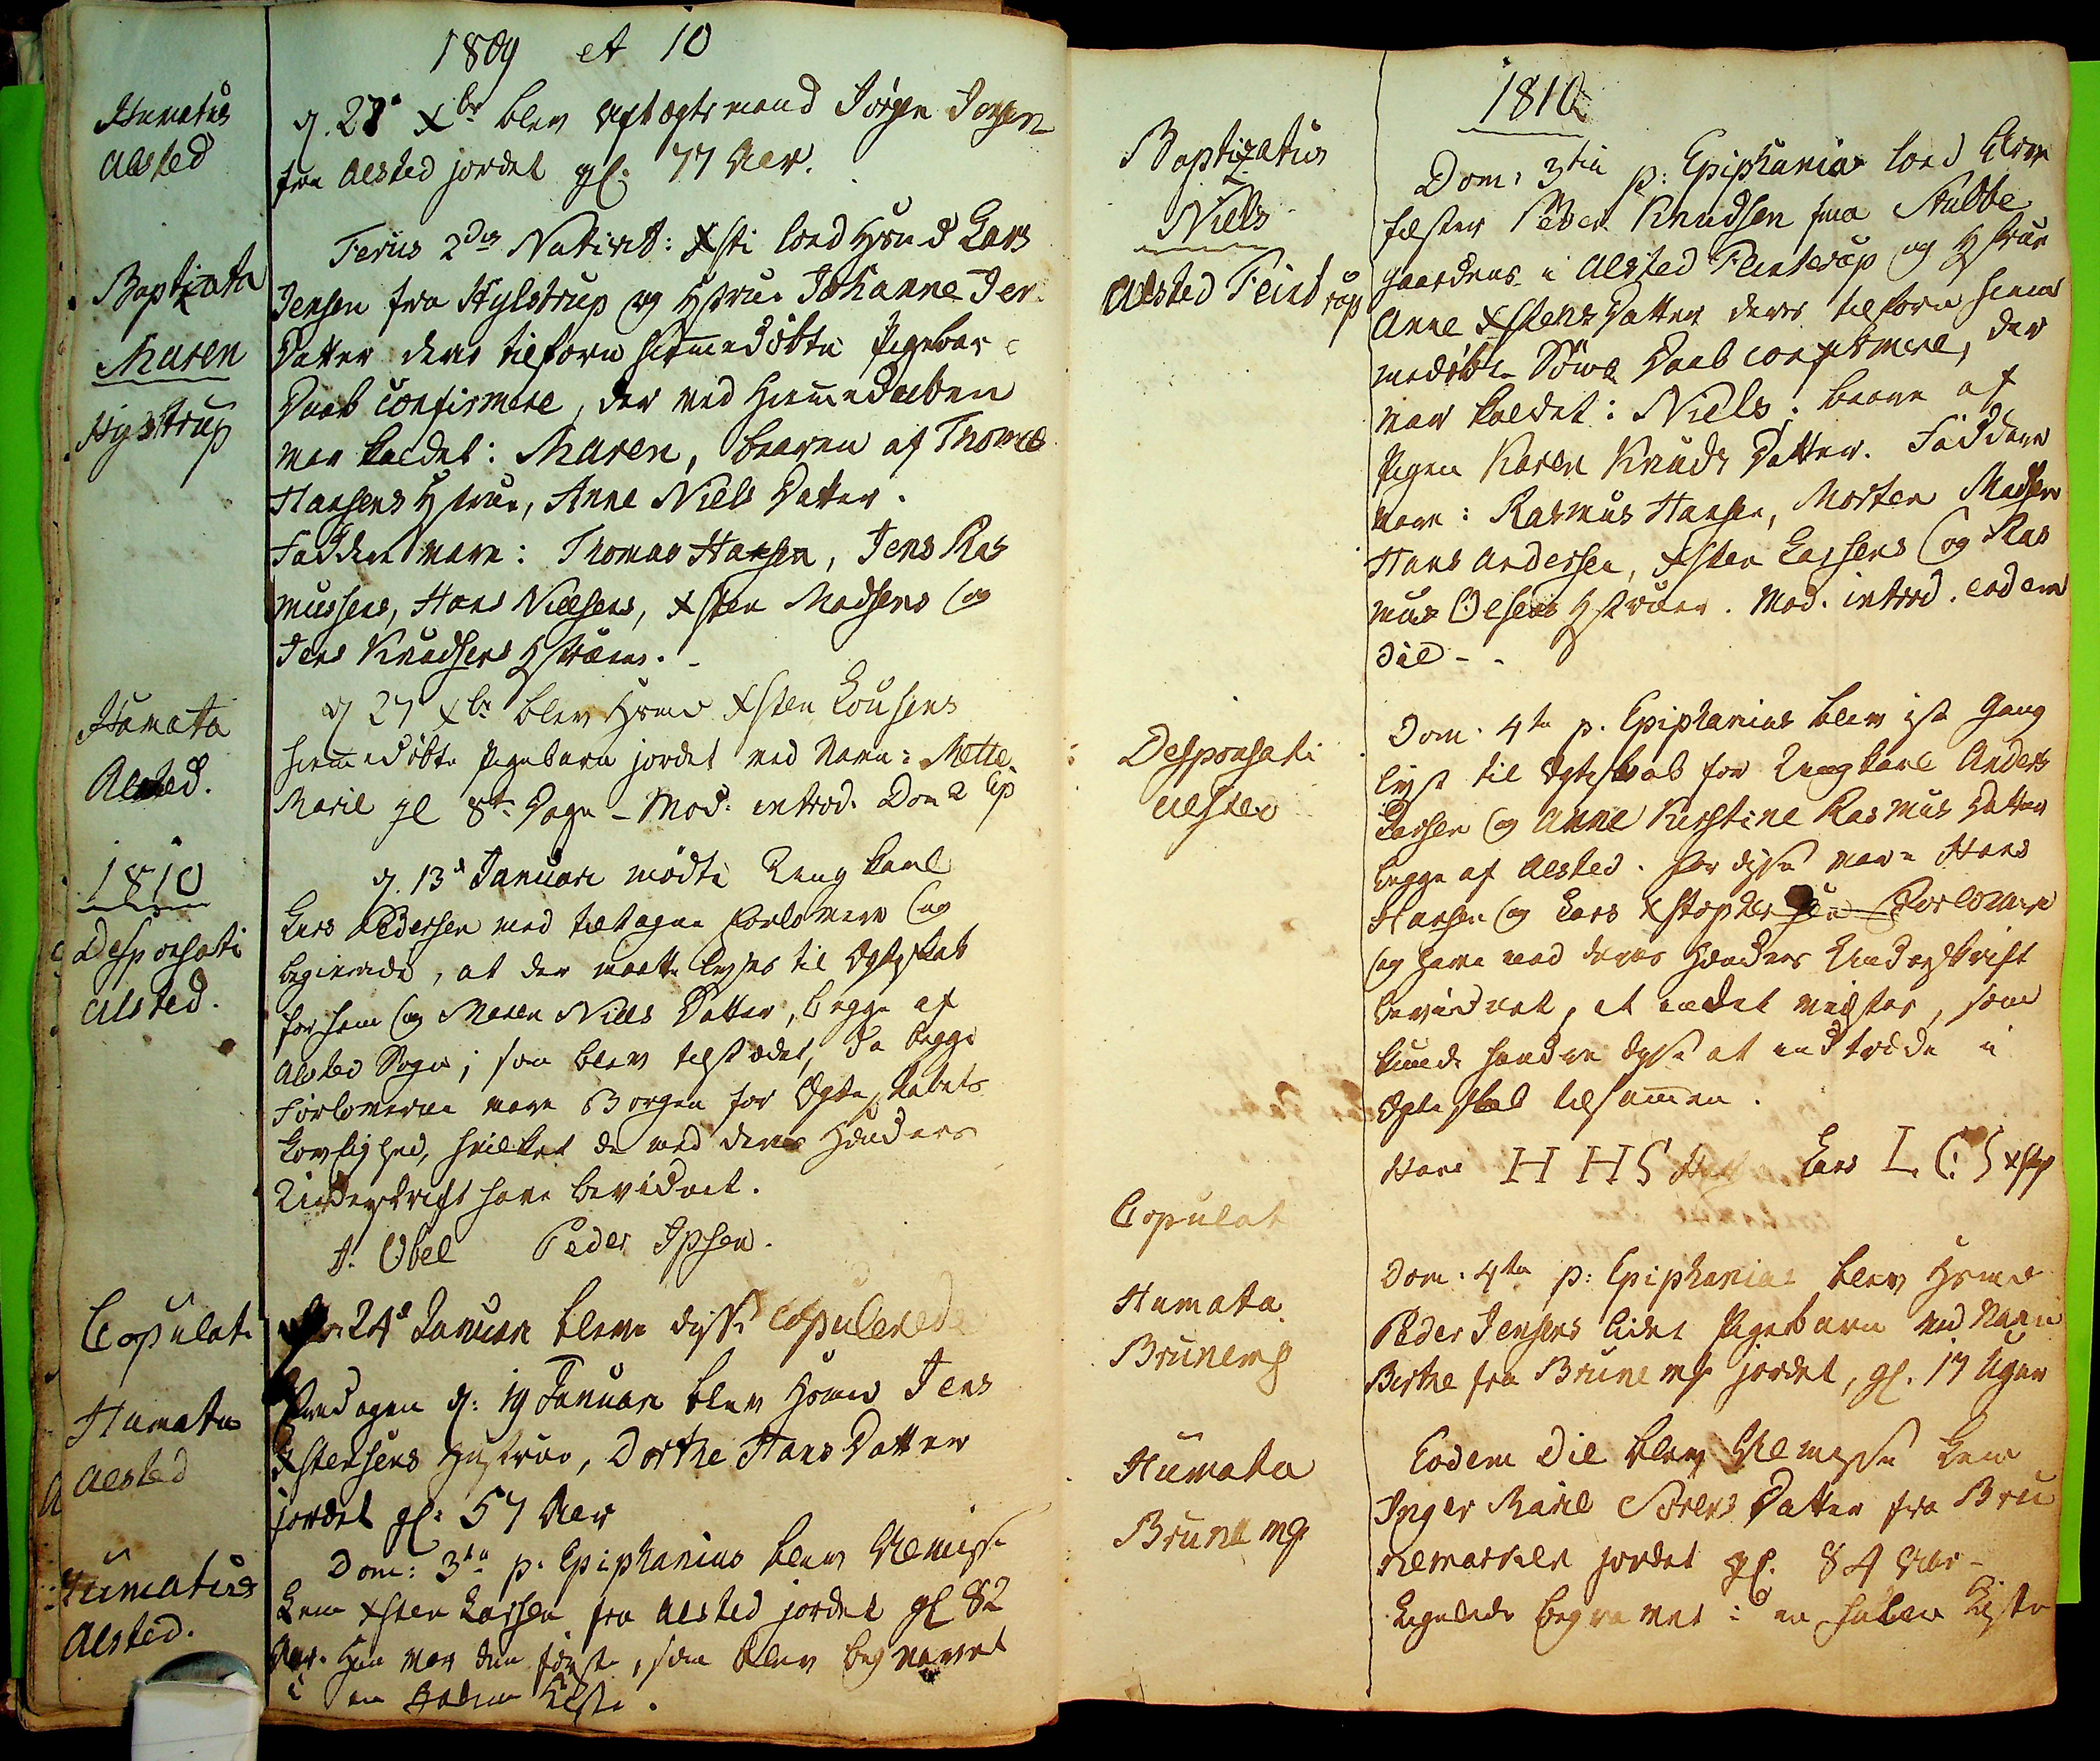Humatus
Alsted
Baptizata
Maren
Hylstrup
Humata
Alsted.
1810
Desponsati
Alsted
Copulati
Humata
Alsted
Humatus
Alsted
1809 et 10
d 27 Xbr blev Aftægtsmand Jørgen Jensen
fra Alsted jordet gl: 77 Aar.
Feriis 2den Nativt: Xsti leod Hsmd Lars
Jensen fra Hylstrup og Hstru Johanne Je##
Datter dere tillforn hiemmedøbte Pigebar##
Daab Confirmere, der ved Hiemmedaaben
var kaldet: Maren, baaren af Thomas
Hansens Hstruye, Anne Niels Datter.
Faddere vare: Thomas Hansen, Jens Ras¬
mussens, Hans Nielsens, Xsten Madsens og
Jens Knudsens Hstruer.
d 27 Xbr blev Hsmd Xsten Lousens
hiemedøbte Pigebarn jordet ved Navn: Mette
Marie gl 8te Dage - Mod: introd: Dom 2 Ep
d. 13de Januari mødte Ung m Karl
Lars Pedersen ved tiltagne Forlovere og
begierde, at der uægte lyses til Ægteskab
for ham og Maren Niels Datter, begge af
Alsted Sogn; som blev tilstædet da begge
Forlovere vare Borgen for Ægteskabets
Lovlighed, hvilket de ved dene Hænders
Underskrift have bevidnet.
J. Obel Peder Ipsen
## 24## Januar bleve disse copulerede
Fredagen d: 19 Januar blev Hsmd Jens¬
Xstensens Hustrue, Dorthe Hans Datter
Jordet gl: 57 Aar.
Dom: 3ta p. Epiphanias blev Almisse
Lem Xsten Larsen fra Alsted jordet gl 82
Aar Han den den første, som blev begravet
i en Ha## Kiste.
Baptizatus
Niels
Alsted Flinterup
Desponsati
Alsted
Copulat
Humata
Brunem##
Humata
Brunem##
1810
Dom: 3tia p: Epiphanias loed Arve
fæster Peder Knudsen paa Stubbe
Gaardens i Alsted Flinterup og Hstrue
Anne Xstens Datter deres tilforn hiem
medøbte Søns Daab confirmere der
var kaldet: Niels. baare af
Pigen Karen Knuds Datter. Faddere
vare: Rasmus Hansen, Morten Madsen
Hans Anderssen, Xten Larsens og Ras
mus Olsens Hstruer. Mod. introd: eodem
die -
Dom: 4ta p. Epiphanias blev 1st Gang
lyst til Ægteskab for Ungkarl Anders
Larsen og Anne Kirstine Rasmus Datter
begge af Alsted for disse vare Hans
Hansen og Lars Xstophersen Forlovere
og have ved deres Hænders Underskrift
bevidnet, at ##det vidstes, som
kunde hændre disse at indsræde i
Ægteskab tilsammen
Hans H H S Hansen Lars L C S Xstens
Dom: 4ta p: Epiphanias blev Hsmd
Peder Jensens lidet Pigebarn ved Navn
Birthe fra Brunem## jordet gl. 17 Uger
Eodem Die blev Almisse Lem
Inger Marie Sørens Datter fra Bru
nemarken jordet gl: 84 Aar.
Ligeledes begravet i en hal## Kiste
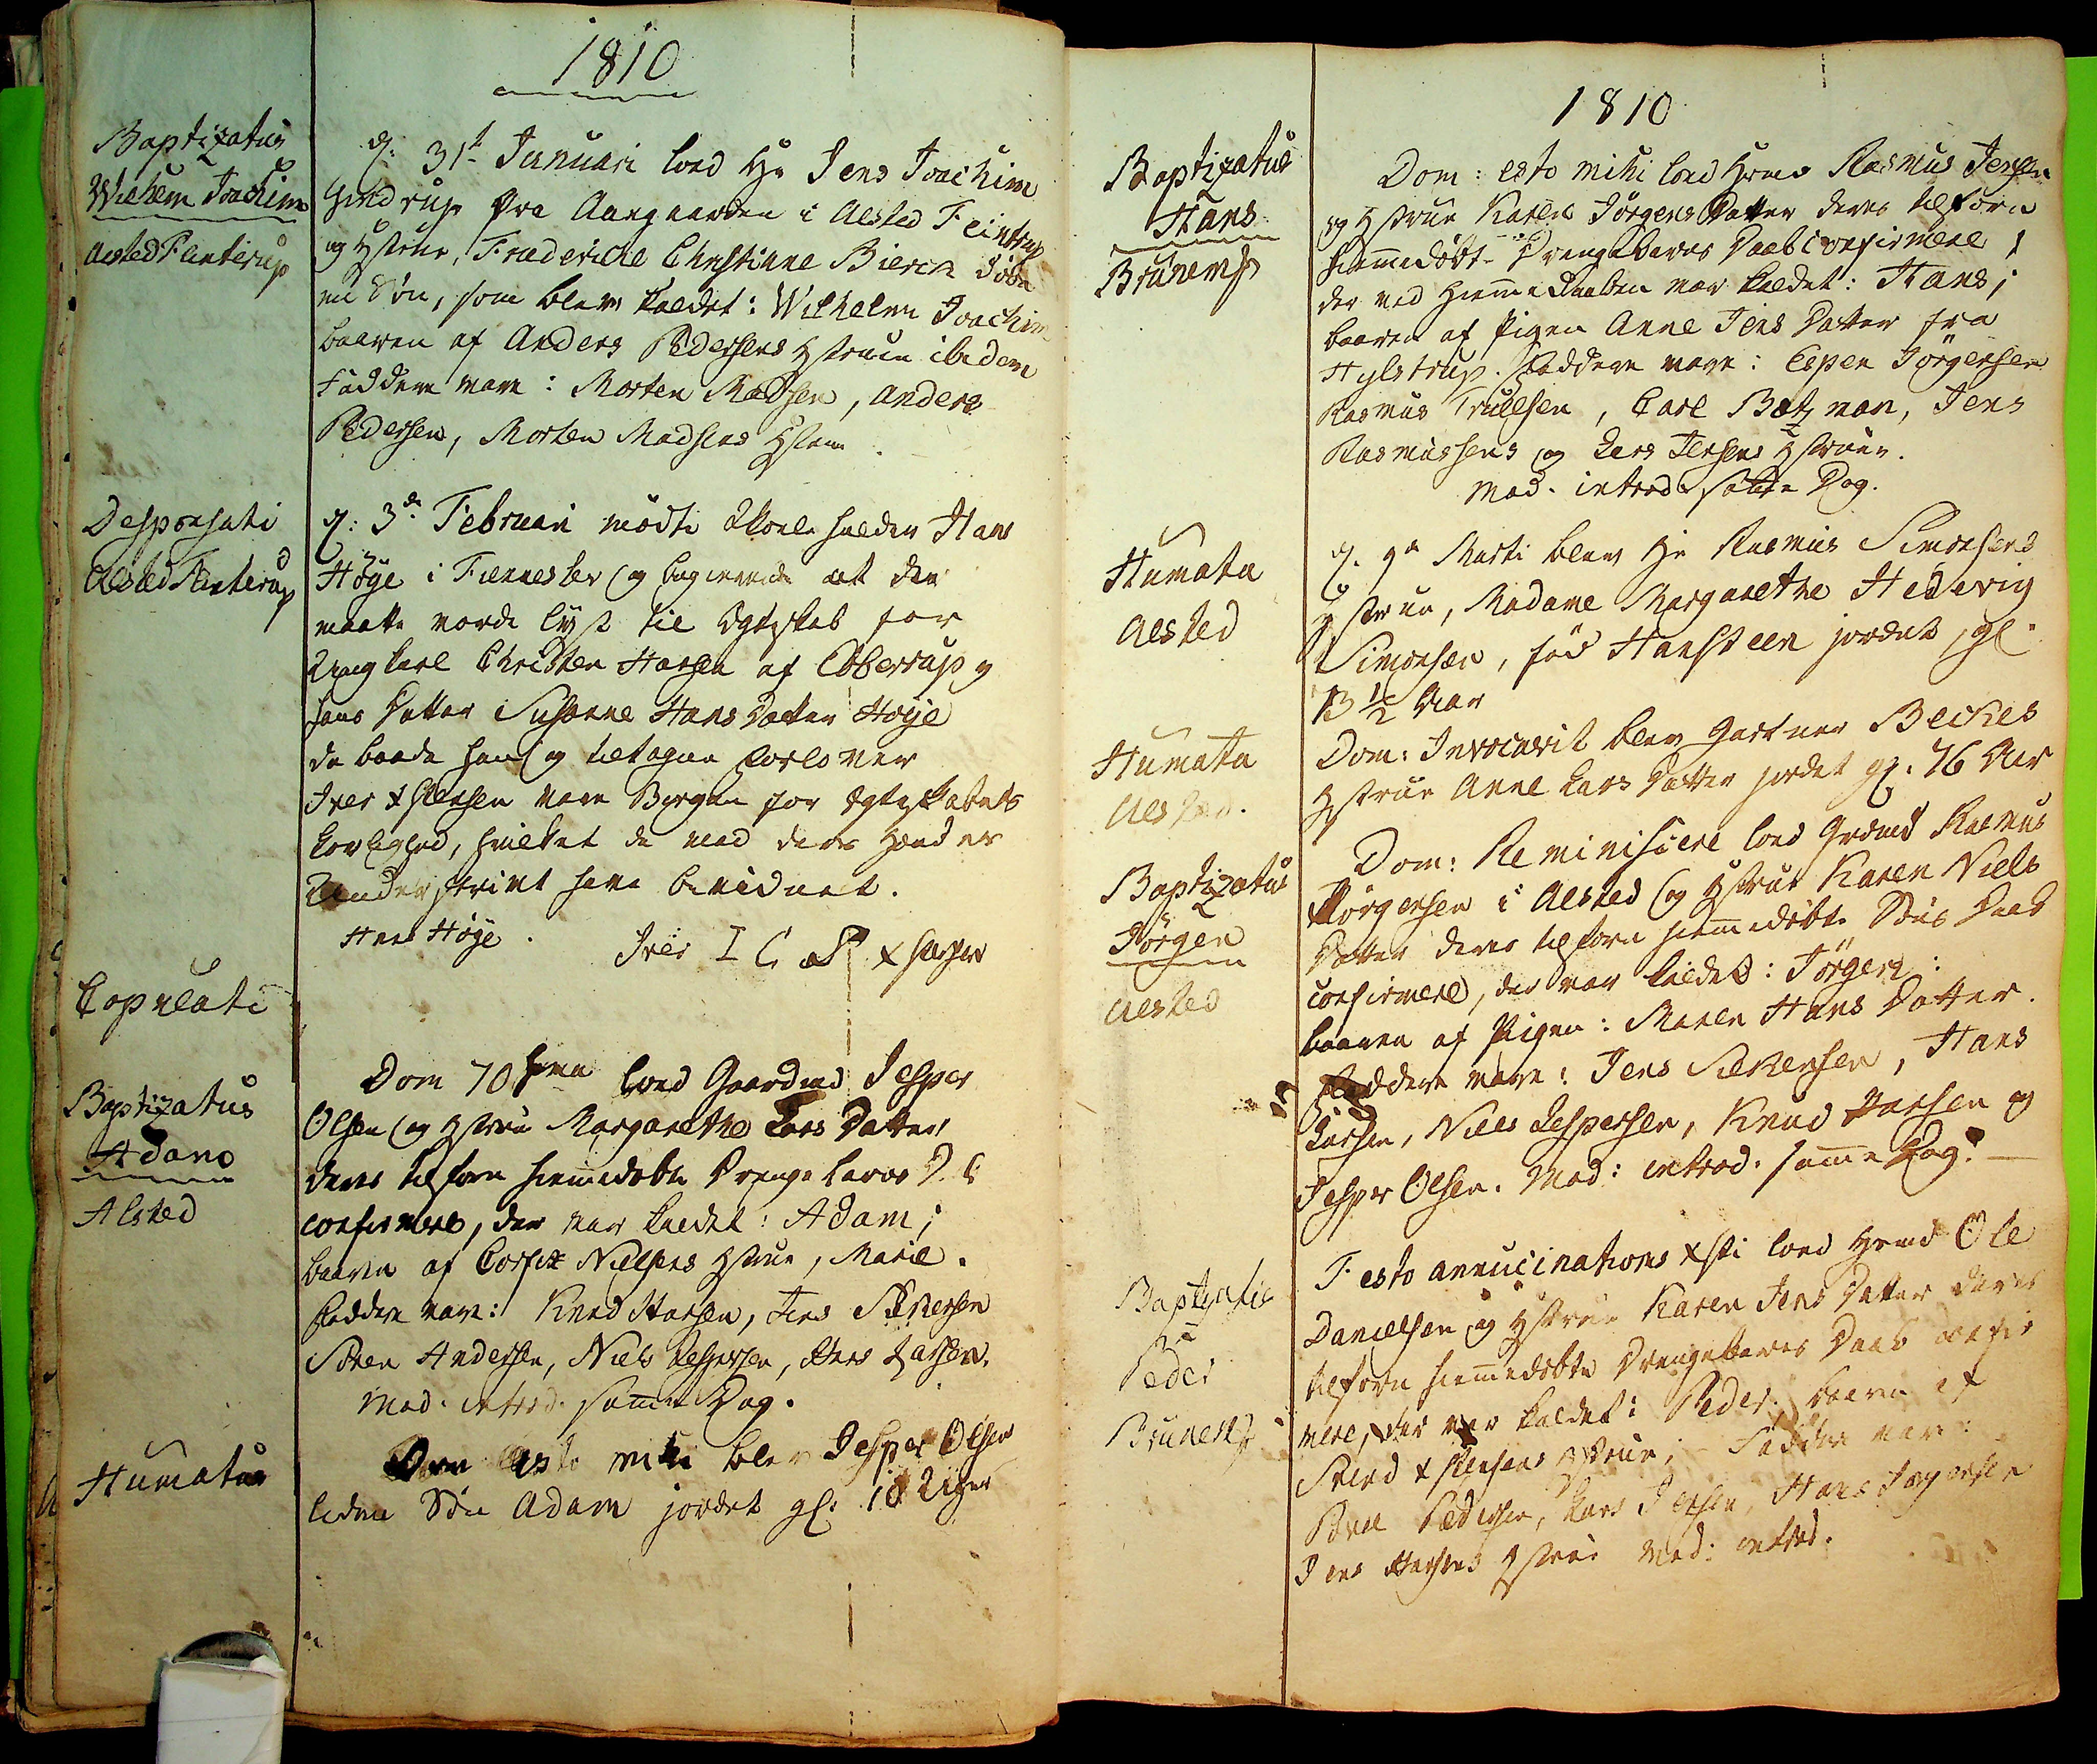Baptizatus
Wilhlm Joachim
Alsted Flinterup
Desponsati
Alsted Flinterup
Copulati
Baptizatus
Adam
Alsted
Humatus
1810
d: 31t Januari loed Hr Jens Joachim
Gindrup fra Aaegaarden i Alsted Flinterup
og Hustrue Frideriche Christiane Bierch døbe
en Søn, som blev kaldet: Wilhelm Joachim
baaren af Anders Pedersens Hstrue ibidem
Faddere vare: Morten Madsen, Anders
Pedersen, Morten Madsens Hstrue
d: 3de Februari mødte Skoeleholder Hans
Høye i Fienneslev og begierede at der
maatte vorde lyst til Ægteskab for
Ungkarl Christen Hansen af Ebberup og
Hans Datter Susanne Hans Datter Høye
da baade hans og tiltagne Forlover
Iver Xstensen vare Borgen for Ægteskabets
Lovlighed, hvilket de ved deres Hændes
Underskrivt have bevidnet
Hans Høge.
Iver I C S Xstensen
Dom 70sima loed Gaardmd Jesper
Olsen og Hstru Margarethe Lars Datter,
deres tilforn hiemmedøbte Drengebarns Daab
cnfirmere, der var kaldet: Adam;
baaren af Corfit Nielsens Hstrue, Marie.
Faddere vare: Knud Hansen, Jens Silkensen
Søren Anderssen, Niels Jespersen, Hans Larsen.
Mod: introd: samme Dag.
Dom: Esto mihi blev Jeppe Olsøn
liden Søn Adam jordet gl: 10 Uger
Baptizatus
Hans
Brunem##
Humata
Alsted
Humata
Alsated.
Baptizatus
Jørgen
Alsted
Baptizatus
Peder
Brunem##
1810
Dom: Esto mihi loed Hsmd Rasmus Jensen
og Hstrue Karen Jørgens Datter deres tilforn
hiemmedøbte Drengebarns Daab confirmere
der ved Hiemmedaaben var kaldet :Hans;
baaren af Pigen Anne Jens Datter fra
Hylstrup. Faddere vare. Espen Jørgensen
Rasmus Truelsen, Carl Bæitzman, Jens
Rasmussens og Lars Jensens Hstruer.
Mod. introd samme Dag.
d. 9de Marti blev Hr Rasmus Simonsens
Hstrue, Madame Margarethe Hedevig
Simonsen fød Hansteen jordet gl
73½ Aar
Dom: Invocavit blev Gartner Beckes
Hstrue Anne Lars Datter jordet gl: 76 Aar
Dom: Reminiscere cons Grdmd Rasmus
Jørgensen i Alsted og Hstrue Karen Niels
Datter deres tilforn hiemmedøbte Søns Daab
confirmere, der var kaldet: Jørgen
baaren af Pigen:Maren Hans Datter
Faddere vare: Jens Silkensen, Hans
Larsen, Niels Jespersen, Knud Hansen og
Jesper Olsen. Mod: introd: samme Dag.
Festo Annucinations Xsti loed Hsmd Ole
Danielsen og Hstrue Karen Jens Datter deres
tilforn hiemmedøbte Drengebarns Daab confir
mere, der var kaldet: Peder baaren af
Svend Xstensen Hstrue; Faddere vare:
Povel Pedersen, Lars Jensen, Hans Jørgensen
Jens Hansens Hstrue Mod introd.